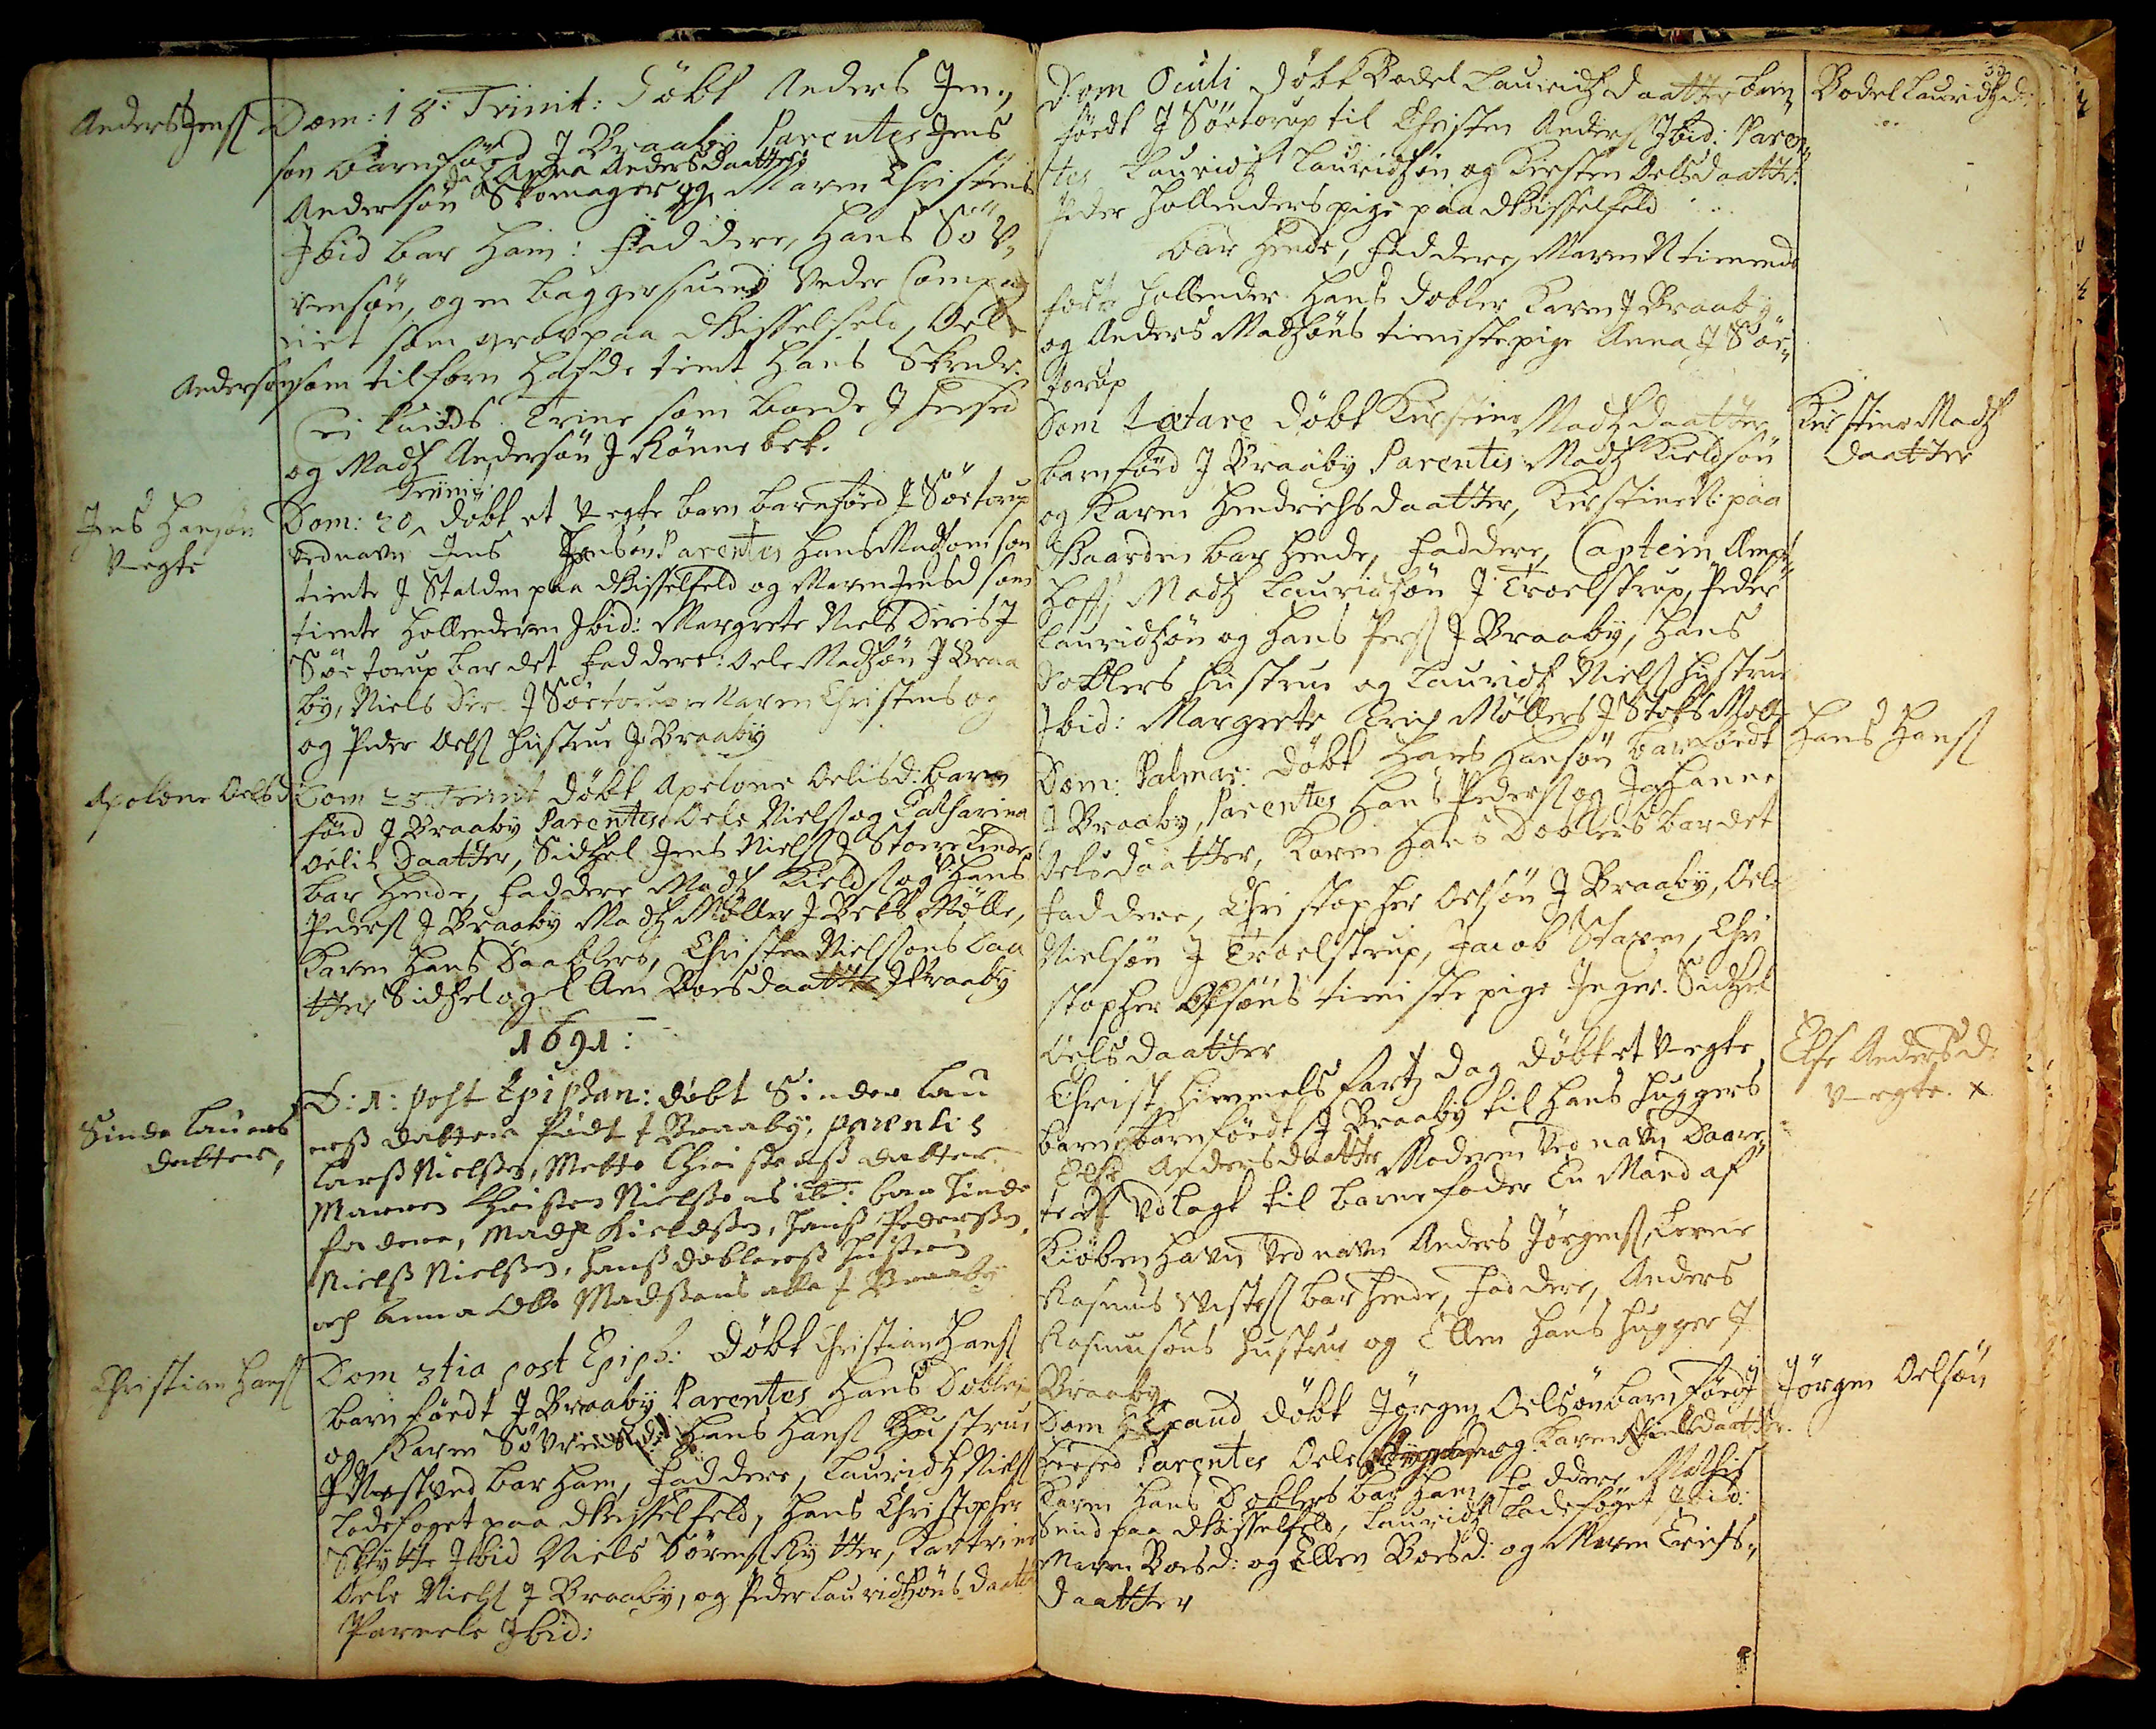Anders Jens
Dom: 18: Trinit: Anders Jen¬
son barnføed J Braaby, Parentes Jens
Anna Andersdaatter
Andersøn Skomager og Maren Christens
Ibid bar Ham: Faddere, Hans Sør¬
rensøn, og en Baggersuend under Compag
niet som grov paa Gisselfeld, Oele
Andersøn som tilforn hafde tient Hans Skredr.
Cei Lunds Trine som boede J Heesed
og Madtz Andersøn J Rønnebek.
Jens Hansøn
Uegte
Triinit:
Dom: 20 døbt et Uegte barn barnføed J Søetorup
ved navn Jens Hansøn. Parentes Hans Madson som 
tiente J Stalden paa Gisselfeld og Maren Jensd som
tiente Hollenderen Jbid: Margrete Niels Diris J
Søe Torup bar det faddere: Oele Madsøn J Braa
by, Niels Dire J Søetorup oc Maren Christens og
og Peder Oels Hustrue J Braaby.
Apolone Oelsd. 
Dom 23. Trinit døbt Apolone Oelisd: barn¬
føed J Braaby Parentes Oele Niels og Catherina
Oelis Daatter, Sidsel Jens Niels J Store Linde
bar Hende, Faddere Madtz Kields og Hans
Peders J Braaby, Madtz Møller J Beks Mølle,
Karen Hans Doeblers, Christen Nielssons Daa
tter Sidtzel og Ellen Boes daatter J Braaby. 
1691: 
Sinde Lauers
datter, 
D: 1: post Epiphan: døbt Sinder Lau
ersdatter født J Braaby, Parentes
Laus Nielssn, Mette Christens datter,
Marren Christen Nielsons ibd: bar Hinde.
fadere, Mads Kieldssn, Hans Pederssn,
Niels Nielssn, Hans Dobleres Hustru
och Anna Olle Madssons alle J Braaby.
Christian Hans
Dom 3tia post Epiph: døbt Christian Hans 
barnføedt J Braaby Parentes Hans Dobler
og Karen Søvrensd: Hans Hans Hustrue
J Nestved bar ham, Faddere, Lauridtz Niels
Ladefoget paa Gisselfeld, Hans Christopher
Skytte Jbid. Niels Sørens Rytter, Kartrine
Oele Niels J Braaby, og Peder Lauridtzøns daatt
Parente Jbid: 
Dom Oculi døbt Bodel Lauridsdaatter barn¬
føedt J Søetorup til Christen Anders Jbid: Paren¬
tes Lauridzs Lauridtsøn og Kirsten Oelsdaatter.
Peder Hollenders pige paa Gisselfeld
bar hende, Faddere, Maren N tienende
for## Hollender. Hans Dobler Karen J Braaby
og Anders Madsøns tienistepige Anna J Søe¬
torup. 
33 
Bodel Lauridtzd. 
Dom Lætare døbt Kirstine Madsdaatter
barn føed J Braaby Parentis Mads Kieldson
og Karen Hendrichsdaatter, Kirstine N: paa
Gaarden bar hende, Faddere, Captein Ampt¬
Hof##. Mads Lauridsøn J Troelstrup, Peder
Lauridsøn og Hans Pers J Braaby, Hans
Doblers Hustrue og Laurids Niels Hustrue
Jbid: Margrete Erich Møllers J Stoks Mølle 
Kirstine Mads
daatter 
Dom: Palmar: døbt Hans Hansøn barnføedt 
J Braaby, Parentes Hans Peders og Johanne
Oelsdaatter, Karen Hans Doblers bar det
Faddere, Christopher Oelsøn J Braaby, Oele
Nielsøn J Troelstrup, Jacob Staxen, Chri
stopher Oelsøns tienistepige Jnger, Sidsel
Oelsdaatter. 
Hans Hans
Christ Himmels Farts dag døbt et Uegte 
barn, barnføedt J Braaby til Hans Huggers 
Else Andersdaatter, Moderen Ved navn Daare¬
te ## udlagt til barnefader En Mand af
Kiøbenhavn ved navn Anders Jørgens, Leene
Rasmus Vestes bar hende, Faddere, Anders
Rasmusøns Hustrue og Ellen Hans Hugger J
Braaby. 
Else Andersd: 
Uegte x
Dom Exaud døbt Jørgen Oelsøn barn føed J 
Heesed, Parentes Oele Jørgensøn## og Karen Nielsdaatter.
Karen Hans Doblers bar ham, Faddere, Mathis
Smid paa Gisselfeld, Laurids Ladefoget Jbid:
Maren Boesd: og Ellen Boesd: og Maren Erichs¬
daatter
Jørgen Oelsøn 
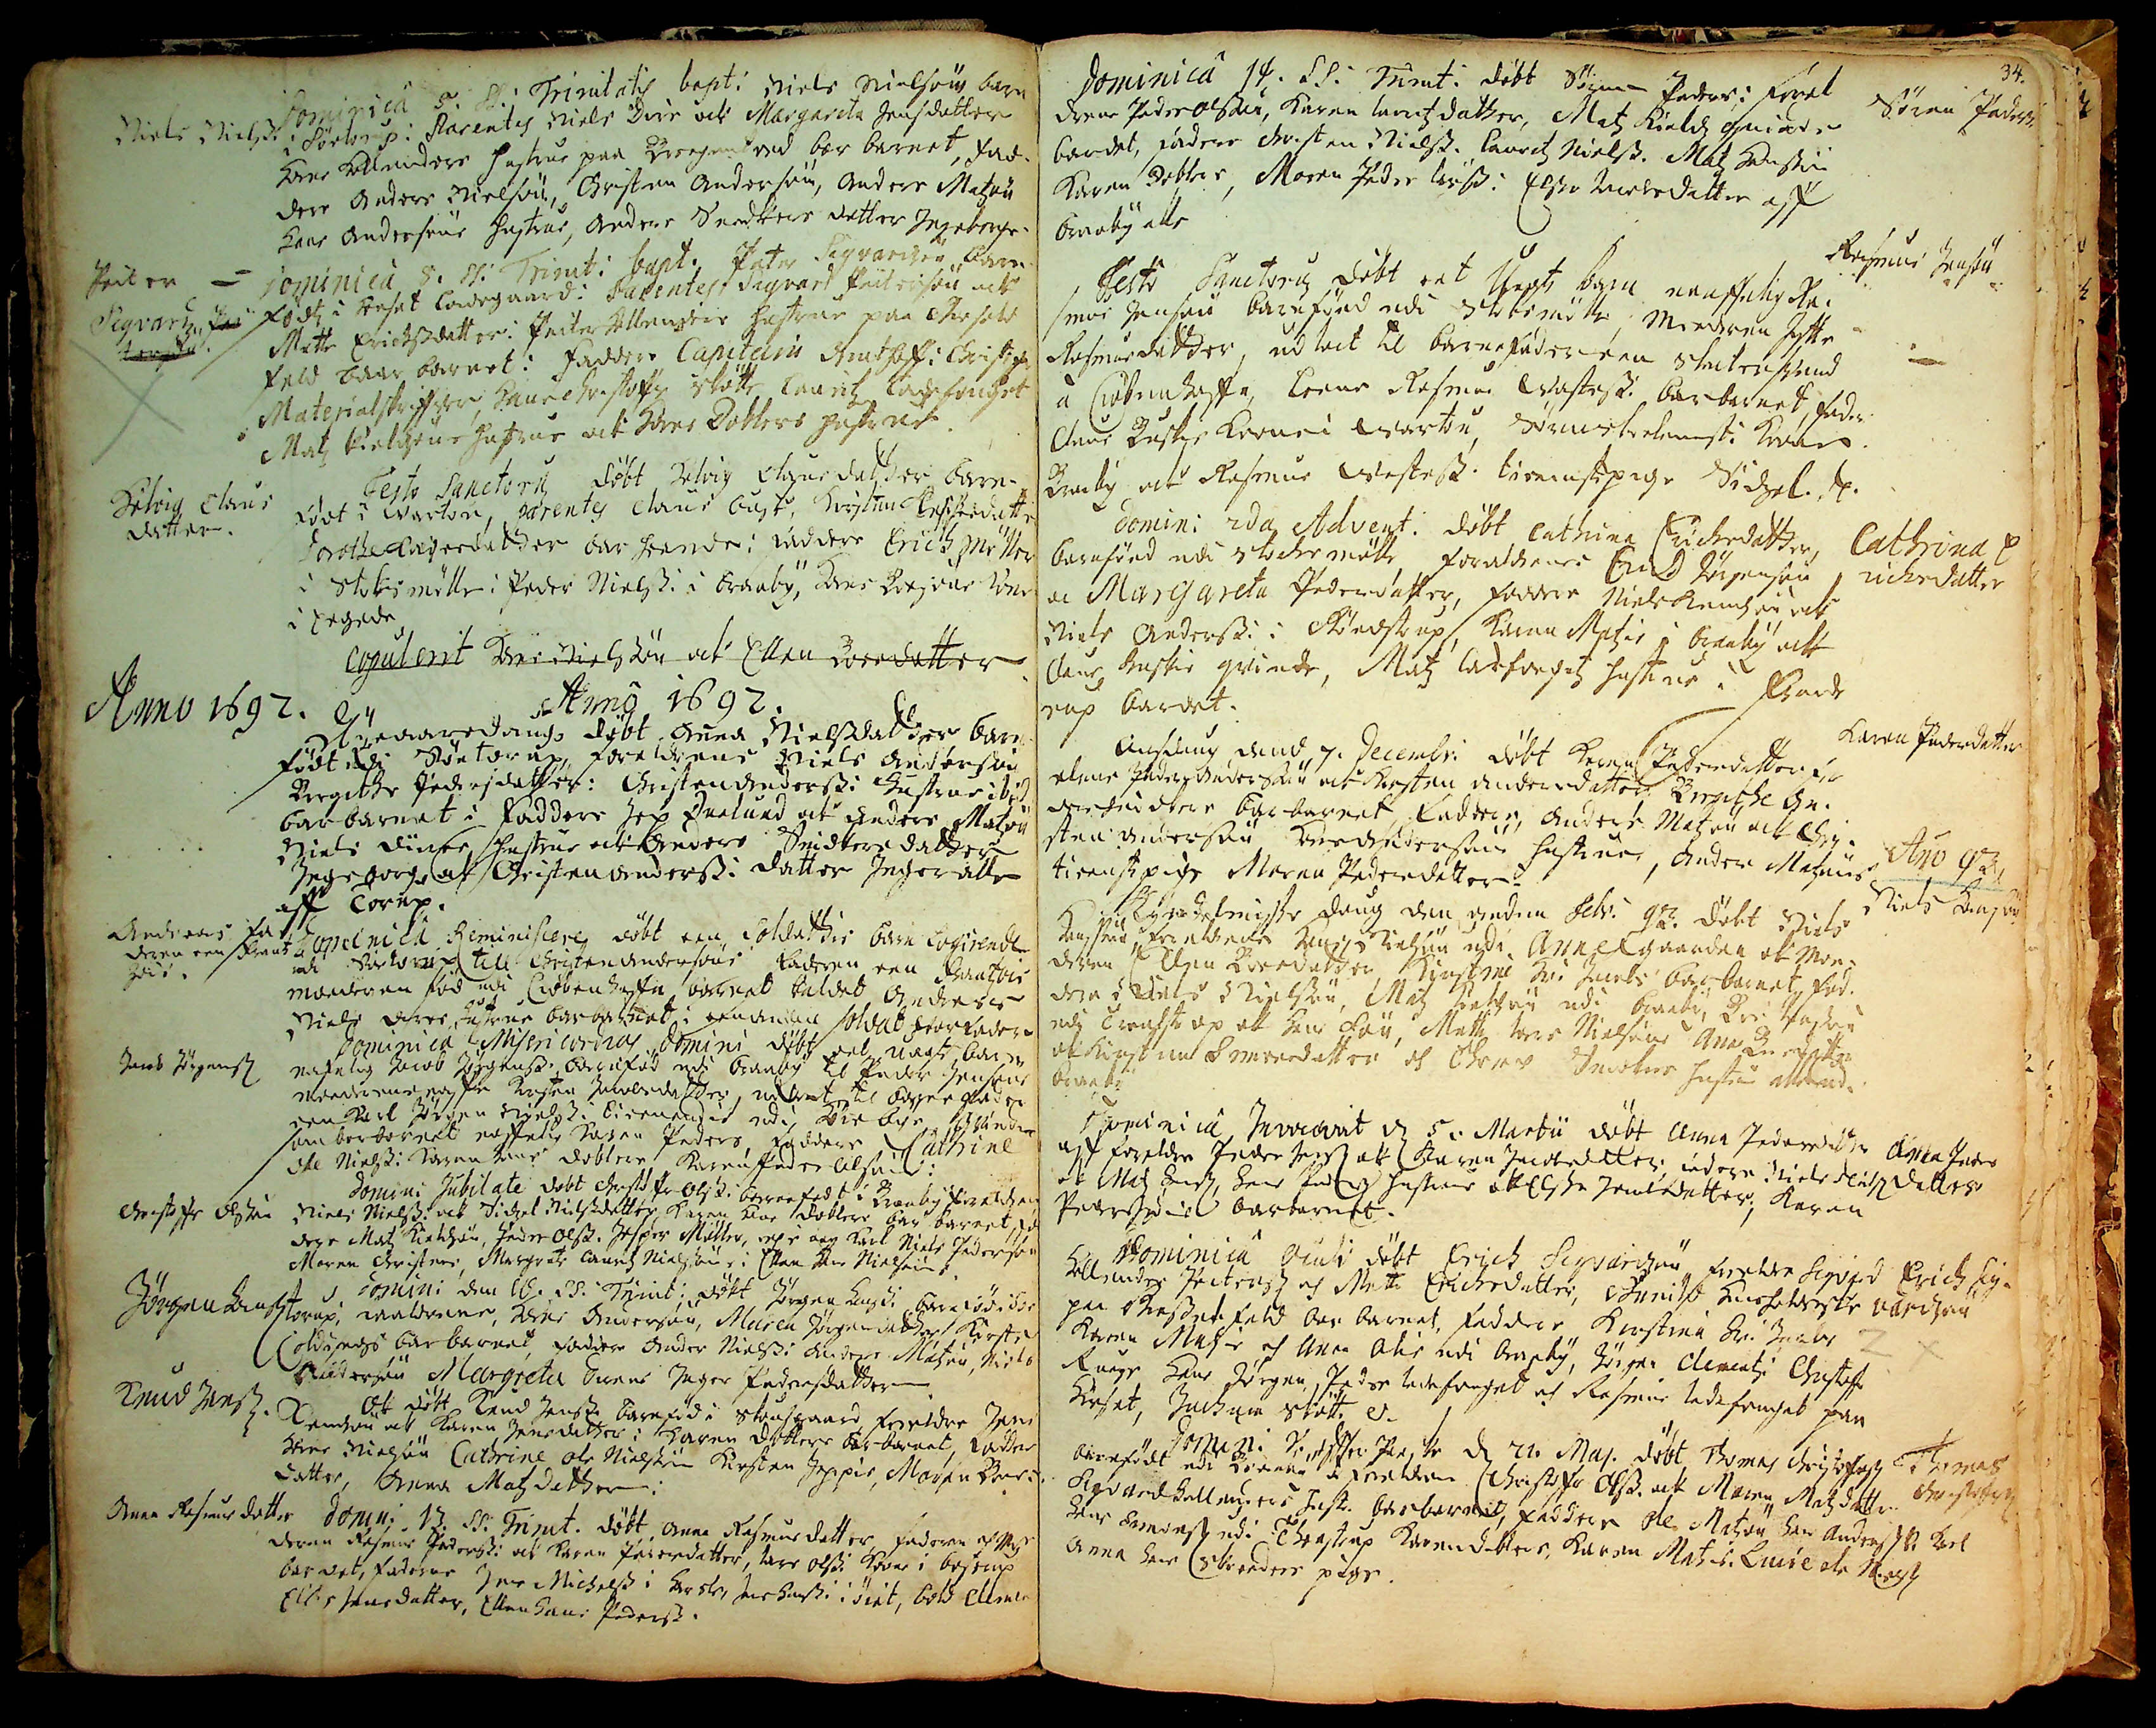Niels Nielsøn 
Dominica 5: SS: Trinitatis bapt: Niels Nielsøn barn 
i Søetorup: Parentes Niels Dire och Margareta Jensdatter
Hans Udriders Hustrue paa Bregentved bar barnet, fad¬
dere. Anders Nielsøn, Christen Andersøn, Anders Matzøn
Hans Andersøns Hustrue, Anders Snedkers datter Ingeborge.
Peiter
Sigvarszn Pet
tersøn
Dominica 8. SS: Trint: bapt. Peter Sigvardsøn barn¬
føed i Heset Ladegaard: Parentes Sigvard Peitersøn och
Mette Erichsdatter: Peiter Albrectes Hustrue paa Giseld
feld bare barnet: Faddere: Capetain Amthoff: Christop.##
Materialskriffver Hanse Kristoffer Skøtte, Lauritz Ladefoeget
Matz Kieldsøns Hustrue och Hans Doblers Hustrue.
Helvig Claus
datter
Festo Sanctorii døbt Helvig Clausdatter barn¬
født i Vartou, Parentes Claus Busk, Kristine Cesstisdattr
Dorothe Oelesdatter bar heende: faddere Erich Møller
i Stoks mølle: Peder Niels i Braaby, Hans Brydes Kone
i Egede. 
Copulerit Hans Nielsøn och Ellen Boesdatter.
Anno 1692 
Anno 1692
Nyeaarsdauge døbt Anna Nielsdaatter barn¬
født udi Søetorup, forældrer Niels Andersøn
Birgithe Pedersdatter: Christen Anders: Hustrue ibid.
bar barnet: Faddere. Jep Duelund och Anders Matzøn
Niels Dines hustrue och Anders Snidkers datter
Ingeborg och Christen Anders: datter Jnger alle
aff Torup.
Andreas Fa
deren een Frent
zois
Dominica Reminisere døbt een Soldathis barn logirende
udi Søetorup till Christen Andersøns, Faderen een Frentzois.
Moederen fød udi Ciøbenhaffn barnet kaldet Andrees
Niels Dires Hustrue bar barnet: Faderne: Soldat zeorfede
Jacob Jørgens
Dominica Misericordia Domini døbt eet uægte barn
nafnlig Jacob Jørgens barnfød udi Braaby til Peder Jensøn
Moderens naffen Kirsten Jacobsdatter. Udlact til barnefader
een Karl Jørgen Niels: tiennendis udi Høiebye, Qvinden
som bar barnet naffnlig Karen Peders, Faddere Cattrine
Ole Niels: Karen Hans Doblers, Karen Peder Olsøns
Christoffer Olsøn 
Domini Jubilate døbt Christoffer Olsøn, barnefødt i Braaby. Forældre
Niels Niels och Sidzel Nielsdatter. Karen Hans doblers bar barnet. Fa¬
dere Matz Kieldsøn, Peder Olss, Jesper Møller, och een Karl Niels Pedersen
Maren Christens, Margrete Laurtz Nielsøns: Ellen Hans Nielsøns.
Jørgen Hans
Domini den 10. SS Trinit: døbt Jørgen Hansøn barnefød i Søe
Torup: foreldrene, Hans Andersøn, Maren Jørgensdatter, Kirsten
Coldings bar barnet, Faddere Ander Nielss: Anders Matzøn, Niels
Andersøn, Margreta Dines, Inger Pedersdatter.
Knud Jensen
Och## døbt Knud Jensøn barnfød i Skousgaard, foreldre, Jens
Svendsøn och Karen Jensdatter: Karen Doblers bar barnet, Faddere
Hans Nielsøn, Cathrine Ole Nielsøns, Kirsten Jeppis, Maren Boes¬
datter, Anna Matzdatter.
Anna Rasmusdatter 
Domini: 13. SS. Trinit. døbt Anna Rasmusdatter, Faderen och Mo
deren Rasmus Peders och Karen Pedersdatter, Lars Ols: Kone i Bosrup
bar det. Fadere: Jens Michels i Hasle Jens Hans i Tiet, bold Clemen
Else Jensdatter, Ellen Hans Peders. 
Dominica 14. SS Trinit døbt Søren Peder: forel
dre Peder Olsøn, Karen Lauritzdatter, Matz Kields quinde
bar det, Fadere Christen Nielss, Lauritz Nielss, Matz Hansøn,
Karen Doblers, Maren Peder Larss, Else Anderdatter aff
Braaby alle.
34.
Søren Pederss
Festo Santorum døbt eet uægte barn nauffnlig Ra¬
smus Jensøn barnføed udi Stoksmølle, Moederen Jytte
Rasmusdatter, udlact til barneFader een slactersvend
i Ciøbenhaffn, Leene Rasmus Vestes: bar barnet, Fadere
Claus Buskis Koene i Vartou, ##### #####
Braaby och Rasmus Vestesens tienestepige Sidzel N.
Rasmus Jensøn 
Domin: 2da Advent: døbt Cathrina Erichsdatter
barnføed udi Stocksmølle, Forældrene Erick Jørgensøn
oc Margareta Pederdatter, Faddere Niels Hansøn och
Niels Anders: i Gøedstrup, Karen Matzis i Braaby och
Claus Buskis qvinde, Matz Ladefoegets Hustrue i Frende
rup bar det. 
Cathrina E¬ 
richsdatter 
Onsdaug dend 7. Decembr: døbt Karen Pedersdatter. for
elere, Peder Anderssøn och Kersten Andersdatter, Bergithe An
ders Snidker bar barnet Faddere, Anders Matzøn ock Chri¬
sten Andersøn, Hans Andersøns Hustrue, Anders Matzøns
tieenste pige Maren Pederdatter: 
Karen Pedersdatter
Anno 93 
Kyendelmisse daug den anden febr 93 døbt Niels
Hanssøn, forældrer Hans Nielsøn udi Annexgaarden ok Moe¬
deren Ellen Boesdatter, Kirstine Hr. Jacobs bar barnet, fad
dere Niels Nielsøn, Matz Keldsøn udi Braaby, Boe Larsøn
udi Troelstrop ok Hans Søn, Mette Lars Nielssøns, Ane Boesdatter
ok Kirsten Sverredatter ok Thomas Vestes Hustru alle udi
Braaby. 
Niels Hansøn 
Dominica Invocavit d. 5. Martii døbt Anna Pedersdatter
aff forelder Peder Jensøn ok Karen Jacobsdatter, Fadere Niels Niels 
ok Matz Hanss, Hans Peders hustrue, ok Else Jacobsdatter, Karen
Peder Jydis bar barnet. 
Anna Peders
datter 
Dominica Oculi: døbt Erich Sigvardsøn, foreldre Sigvard
Hollender Peiters och Mette Erichsdatter, Gunnild Husholderske
paa Gisselfeld bar barnet, faddere Kirstine Hr. Jacobs
Karen Matzis och Ane Olis udi Braaby, Jørgen Clementz, Christoff 
Runge Hans Jørgen, Peder Ladefoeget och Rasmus Ladefoeget paa
Hirset, Jochum Skøtte.
Erich Sig¬
vardsøn 
Domini: V. efter Paaske d 21. Maj døbt Thomas Christoffers
barnfødt udi Braaby a Forældrene. Christoffer Ols ock Maren Matzdatter.
Sigvard Hollenders Hustr. bar barnet, Faddere Ole Matzøn, Hans Anders karl
Hans Sørensen udi Theestrup, Karen Doblers, Karen Matzis, Luice Ole Niels
Anna Hans Skreeders pige. 
Thomas 
Christoffers